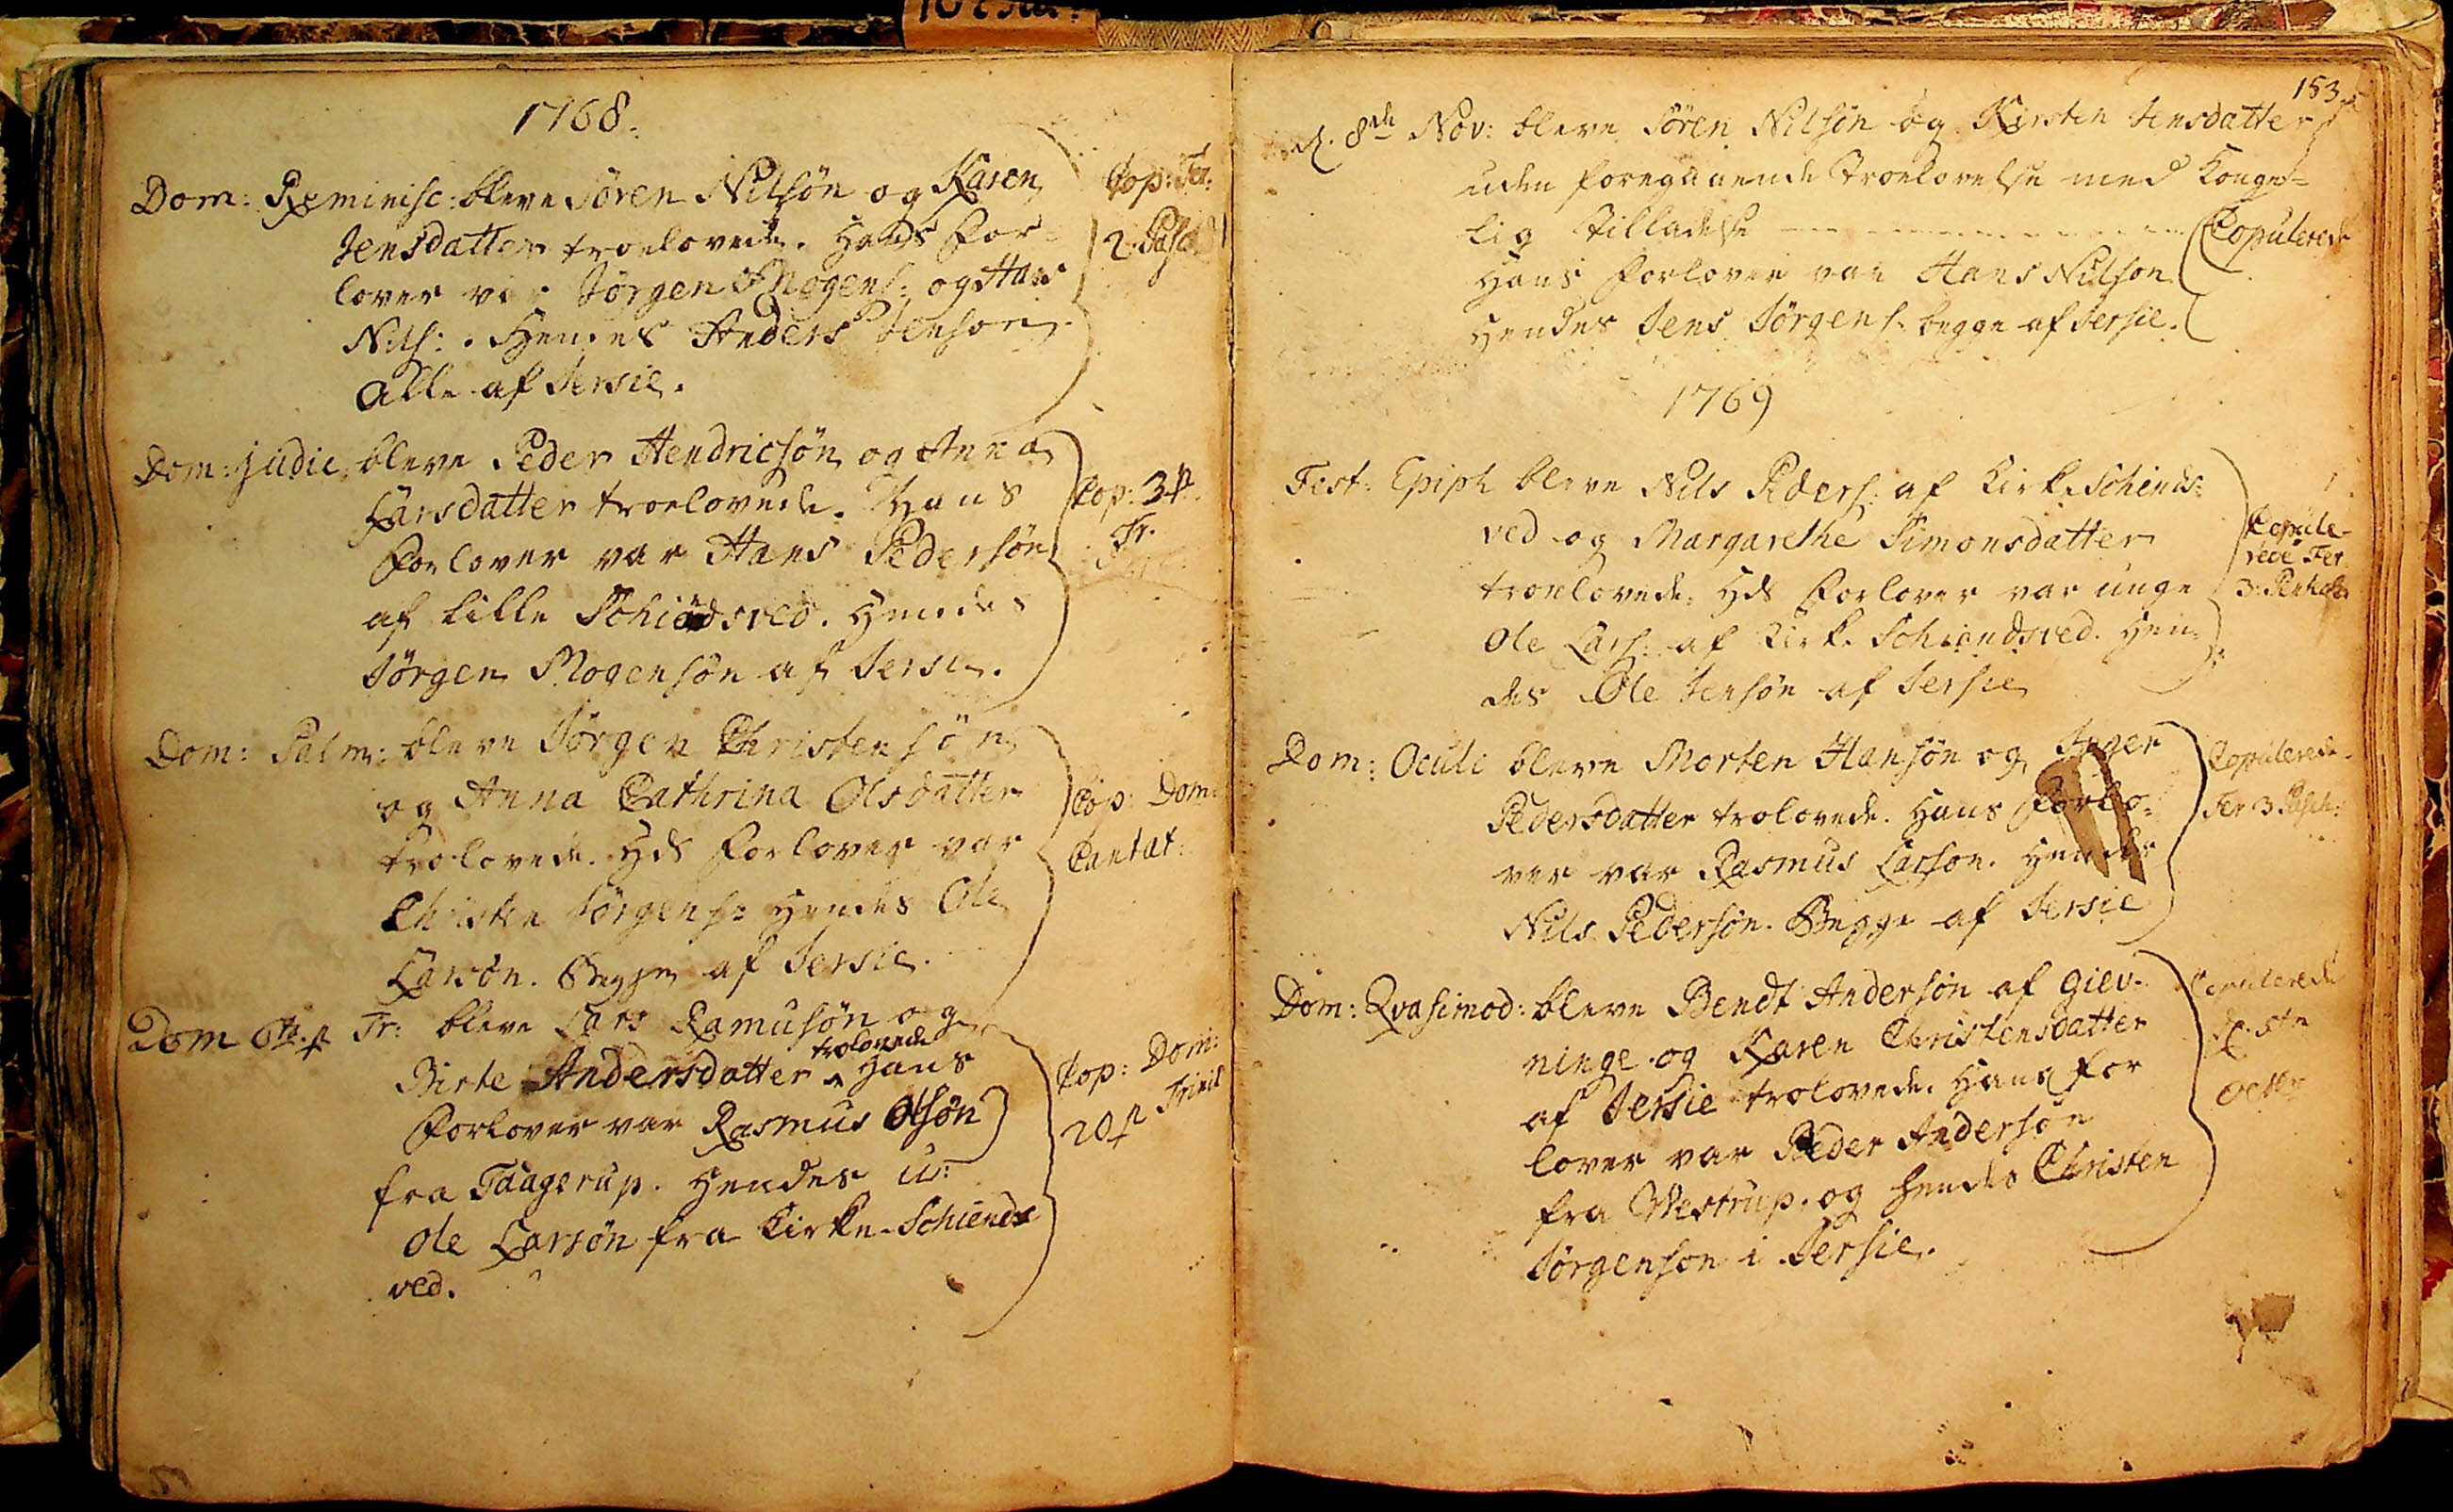1768
Dom: Reminisc: bleve Søren Nilsøn og Karen
Jensdatter troelovede. Hans For¬
lover var Jørgen Mogens: og Hans
Nils:. Hendes Anders Jenson.
Alle af Jersie.
Cop: Fer:
2: Pasc.
Dom: Judic. Bleve Peder Hendricsøn og Anna
Larsdatter troelovede. Hans
Forlover var Hans Pedersøn
af lille Schiendsved. Hendes
Jørgen Mogensøn af Jersie.
Cop: 3 p
Tr.
Dom: Palm: bleve Jørgen Christensøn
og Anna Cathrina Olsdatter
troelovede. Hds Forlover var
Christen Jørgens: Hendes Ole
Larsøn. Begge af Jersie.
Cop: Dom:
Cantat:
Dom 6te. p Tr: bleve Lars Rasmusøn og
troelovede
Birte Andersdatter ^ Hans
Forlover var Rasmus Olsøn
fra Taagerup. Hendes u:
Ole Larsøn fra Kirke-Schiends
ved.
Cop: Dom:
20 p Trinit
153.
d. 8de Nov: bleve Søren Nilsøn og Kirsten Jensdatter
uden foregaaende Troelovelse med Konge¬
lig Tilladelse
Hans Forlover var Hans Nilson
Hendes Jens Jørgens. begge af Jersie.
Copulerede
1769
Fest. Epiph bleve Nils Peders: af Kirke Schinds¬
ved og Margarethe Simonsdatter
troelovede: Hds Forlover var unge
Ole Lars: af Kirke Schiendsved. Hen¬
des Ole Jensøn af Jersie
Copule¬
rede Fer.
3: Pentech:
Dom: Oculi bleve Morten Hansøn og Inger
Pedersdatter trolovede. Hans Forlo¬
ver var Rasmus Larson. Hendes
Nils Pedersøn. Begge af Jersie
Copulerede
Fer 3 Pasch:
Dom: Qvasimod: bleve Bendt Andersøn af Giev¬
ninge og Karen Christensdatter
af Jersie trolovede. Hans For¬
lover var Peder Andersøn
fra Westrup. og hendes Christen
Jørgenson i Jersie.
Copulerede
d 5te
Octbr
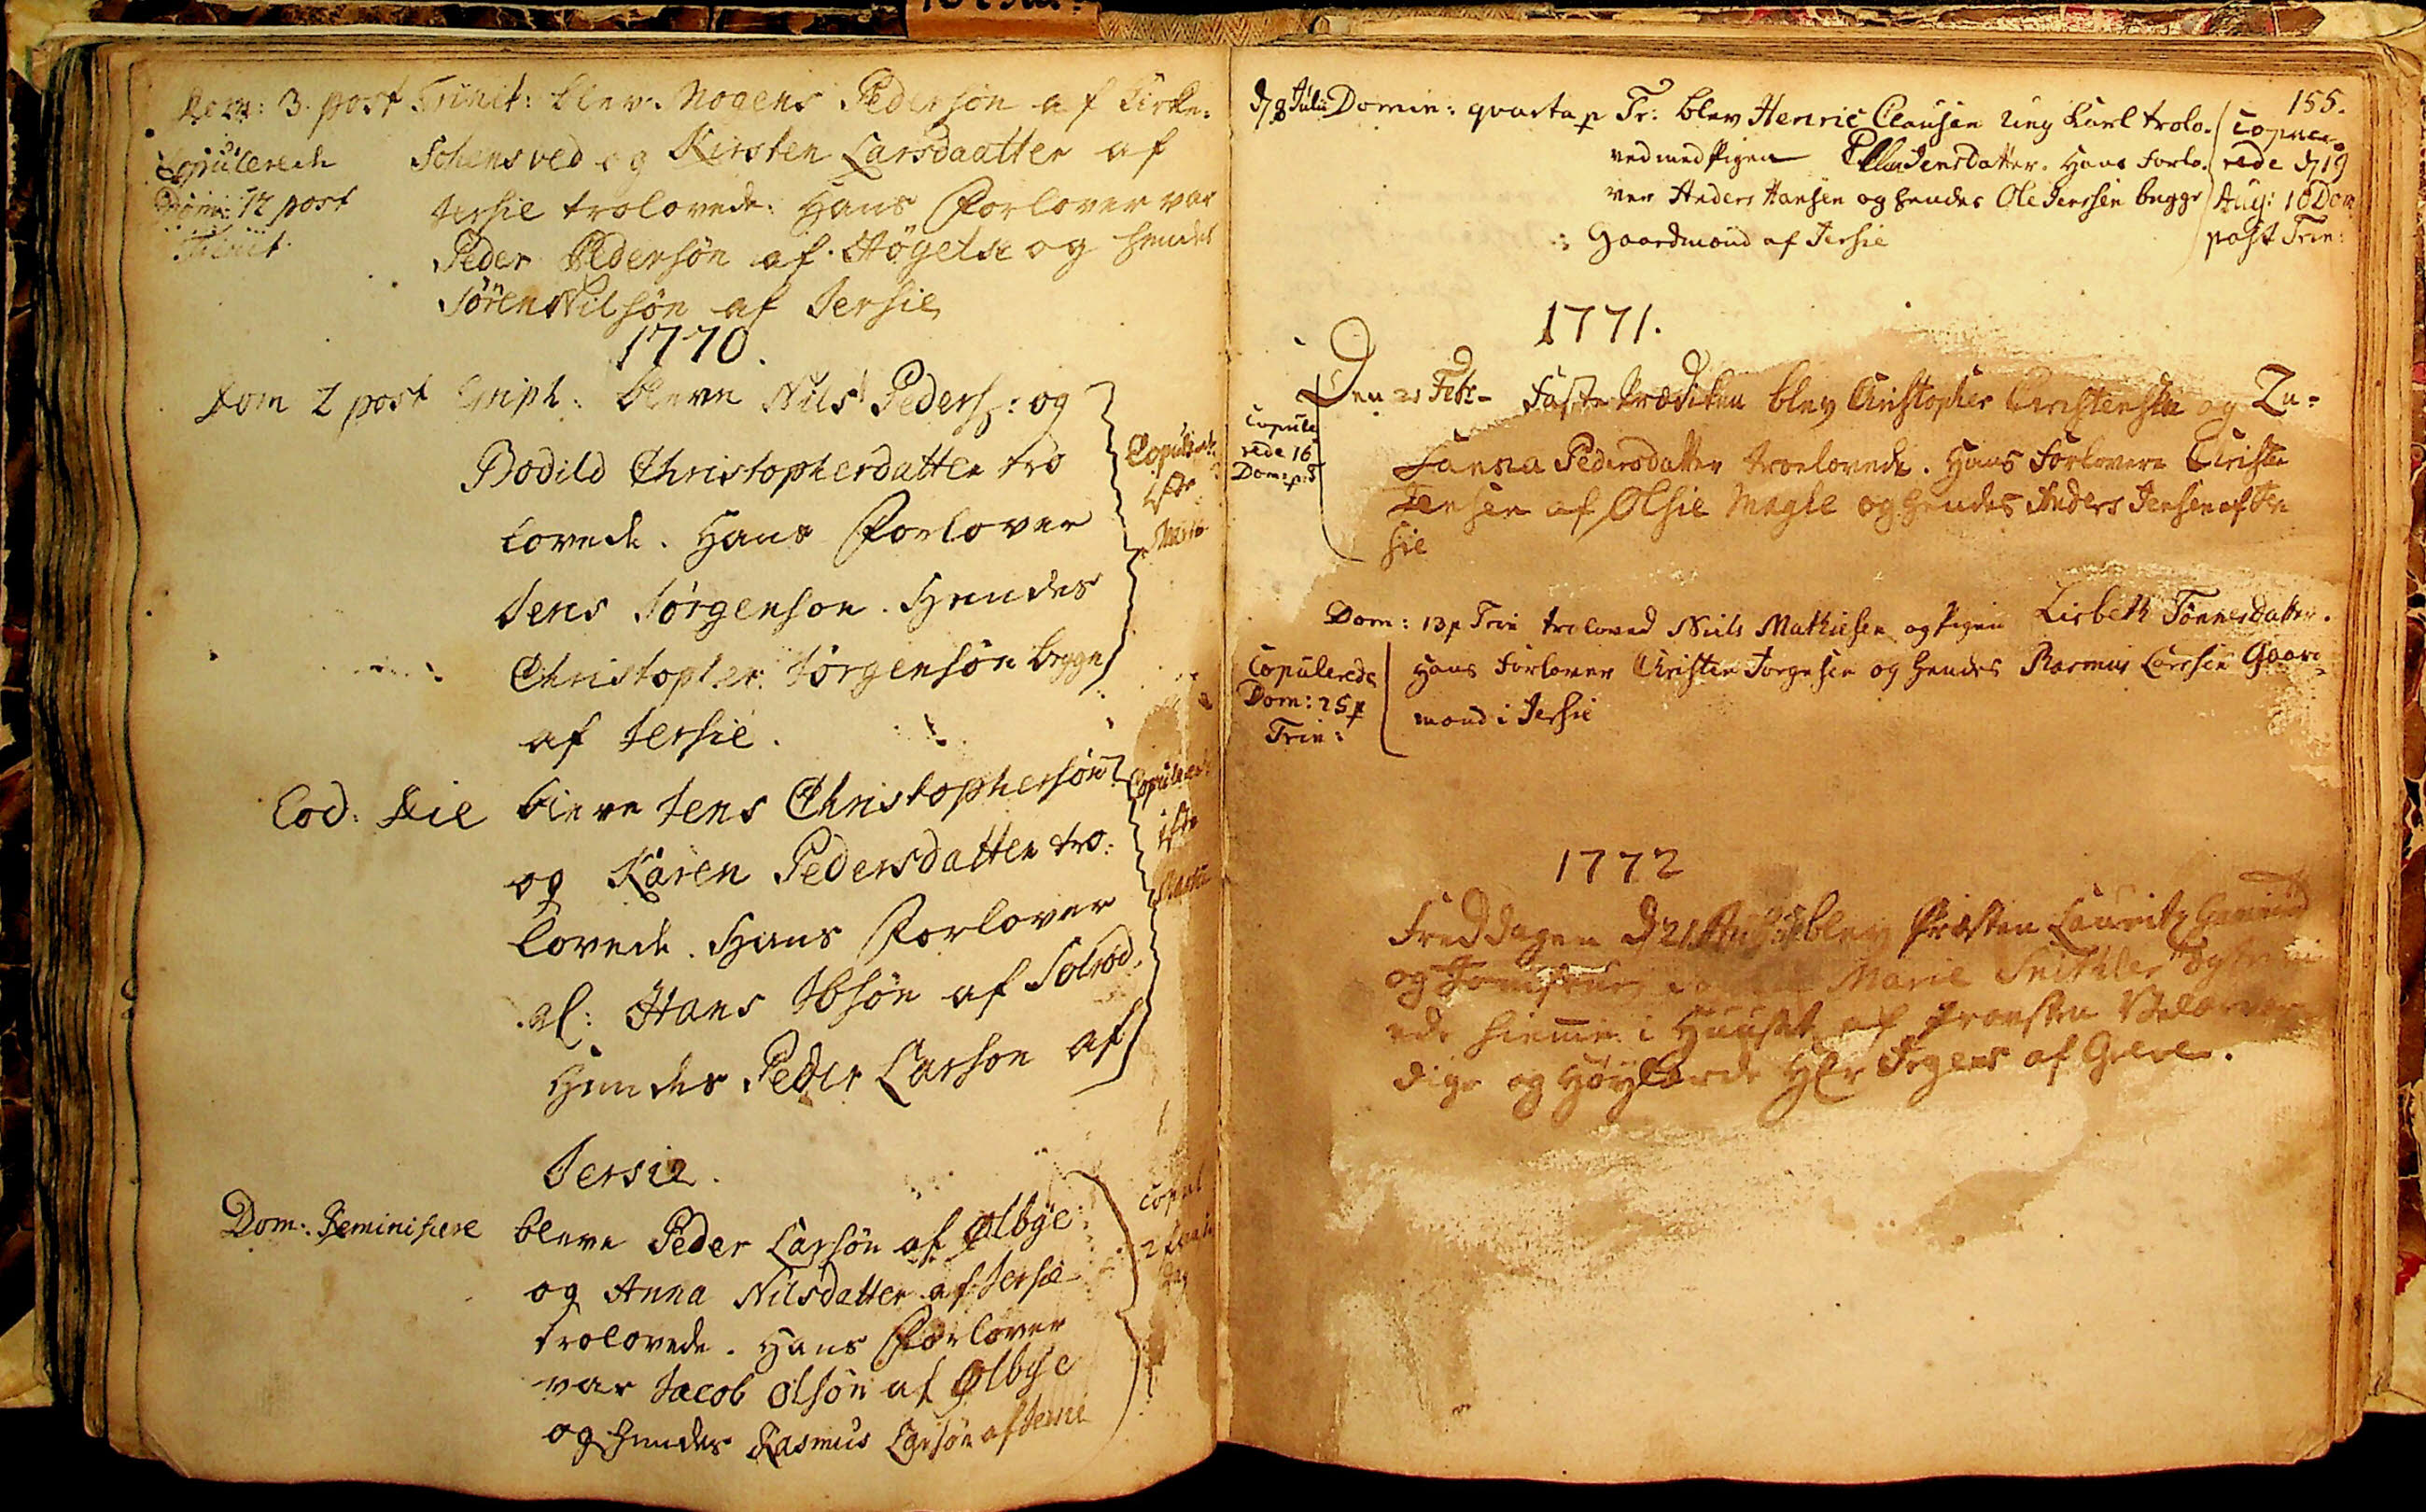Dom: 3. post Trinit: blev Mogens Pedersøn af Kirke¬
Schensved og Kirsten Larsdatter af
Jersie trolovede. Hans Forlover var
Peder Pedersøn af Høgelse og hendes
Søren Nilsøn af Jersie
Copulerede
Dom: 12 post
Trinit
1770.
Dom 2 post Epiph: bleve Nils Pederss: og
Bodild Christophersdatter tro
lovede. Hans Forlover
Jens Jørgensøn. Hendes
Christopher Jørgensøn begge
af Jersie.
Copulerede
1ste
Martii
Eod: Die bleve Jens Christophersøn
og Karen Pedersdatter tro¬
lovede. Hans Forlover
gl: Hans Ibsøn af Solrød.
Hendes Peder Larson af
Jersie.
Copulerede
1ste
Martii
Dom: Reminiscere bleve Peder Larsøn af Ølbye
og Anna Nilsdatter af Jersie
Trolovede. Hans Forlover
var Jacob Olsøn af Ølbye
og hendes Rasmus Larsøn af Jersie
Copul
2 Paaske
Dag
155.
d 8 Julii Domin: qvarta p Tr: blev Henric Clausen Ung Karl trolo¬
ved med Pigen Ellen Jensdatter. Hans Forlo¬
ver Anders Hansen og hendes Ole Jenssen begge
Gaardmænd af Jersie
Copule¬
rede d 19
Aug: 10 Dom
post Trin:
1771.
Den 21 Febr. Faste Prædiken blev Christopher Christensen og Zu¬
sanna Pedersdatter troelovede. Hans Forlovere Christen
Jensen af Ølsie Magle og Hendes Anders Jensen af Jer¬
sie
Copule¬
rede 16
Dom: p: T
Dom: 13 p Trin troloved Niels Mathiesen og Pigen Lisbeth Tønnes Datter.
Hans Forlover Christen Jørgnsen og Hendes Rasmus Larssen Gaard¬
mænd i Jersie
Copulerede
Dom: 25 p
Trin:
1772
Fredagen d 21 Aug blev Præsten Lauritz Gammerød
og Jomfrue Sophia Marie Snithler ægtevi
ede hiemme i Huuset af Præsten Velærvær
dige og Høylærde Hr Irgens af Greve.